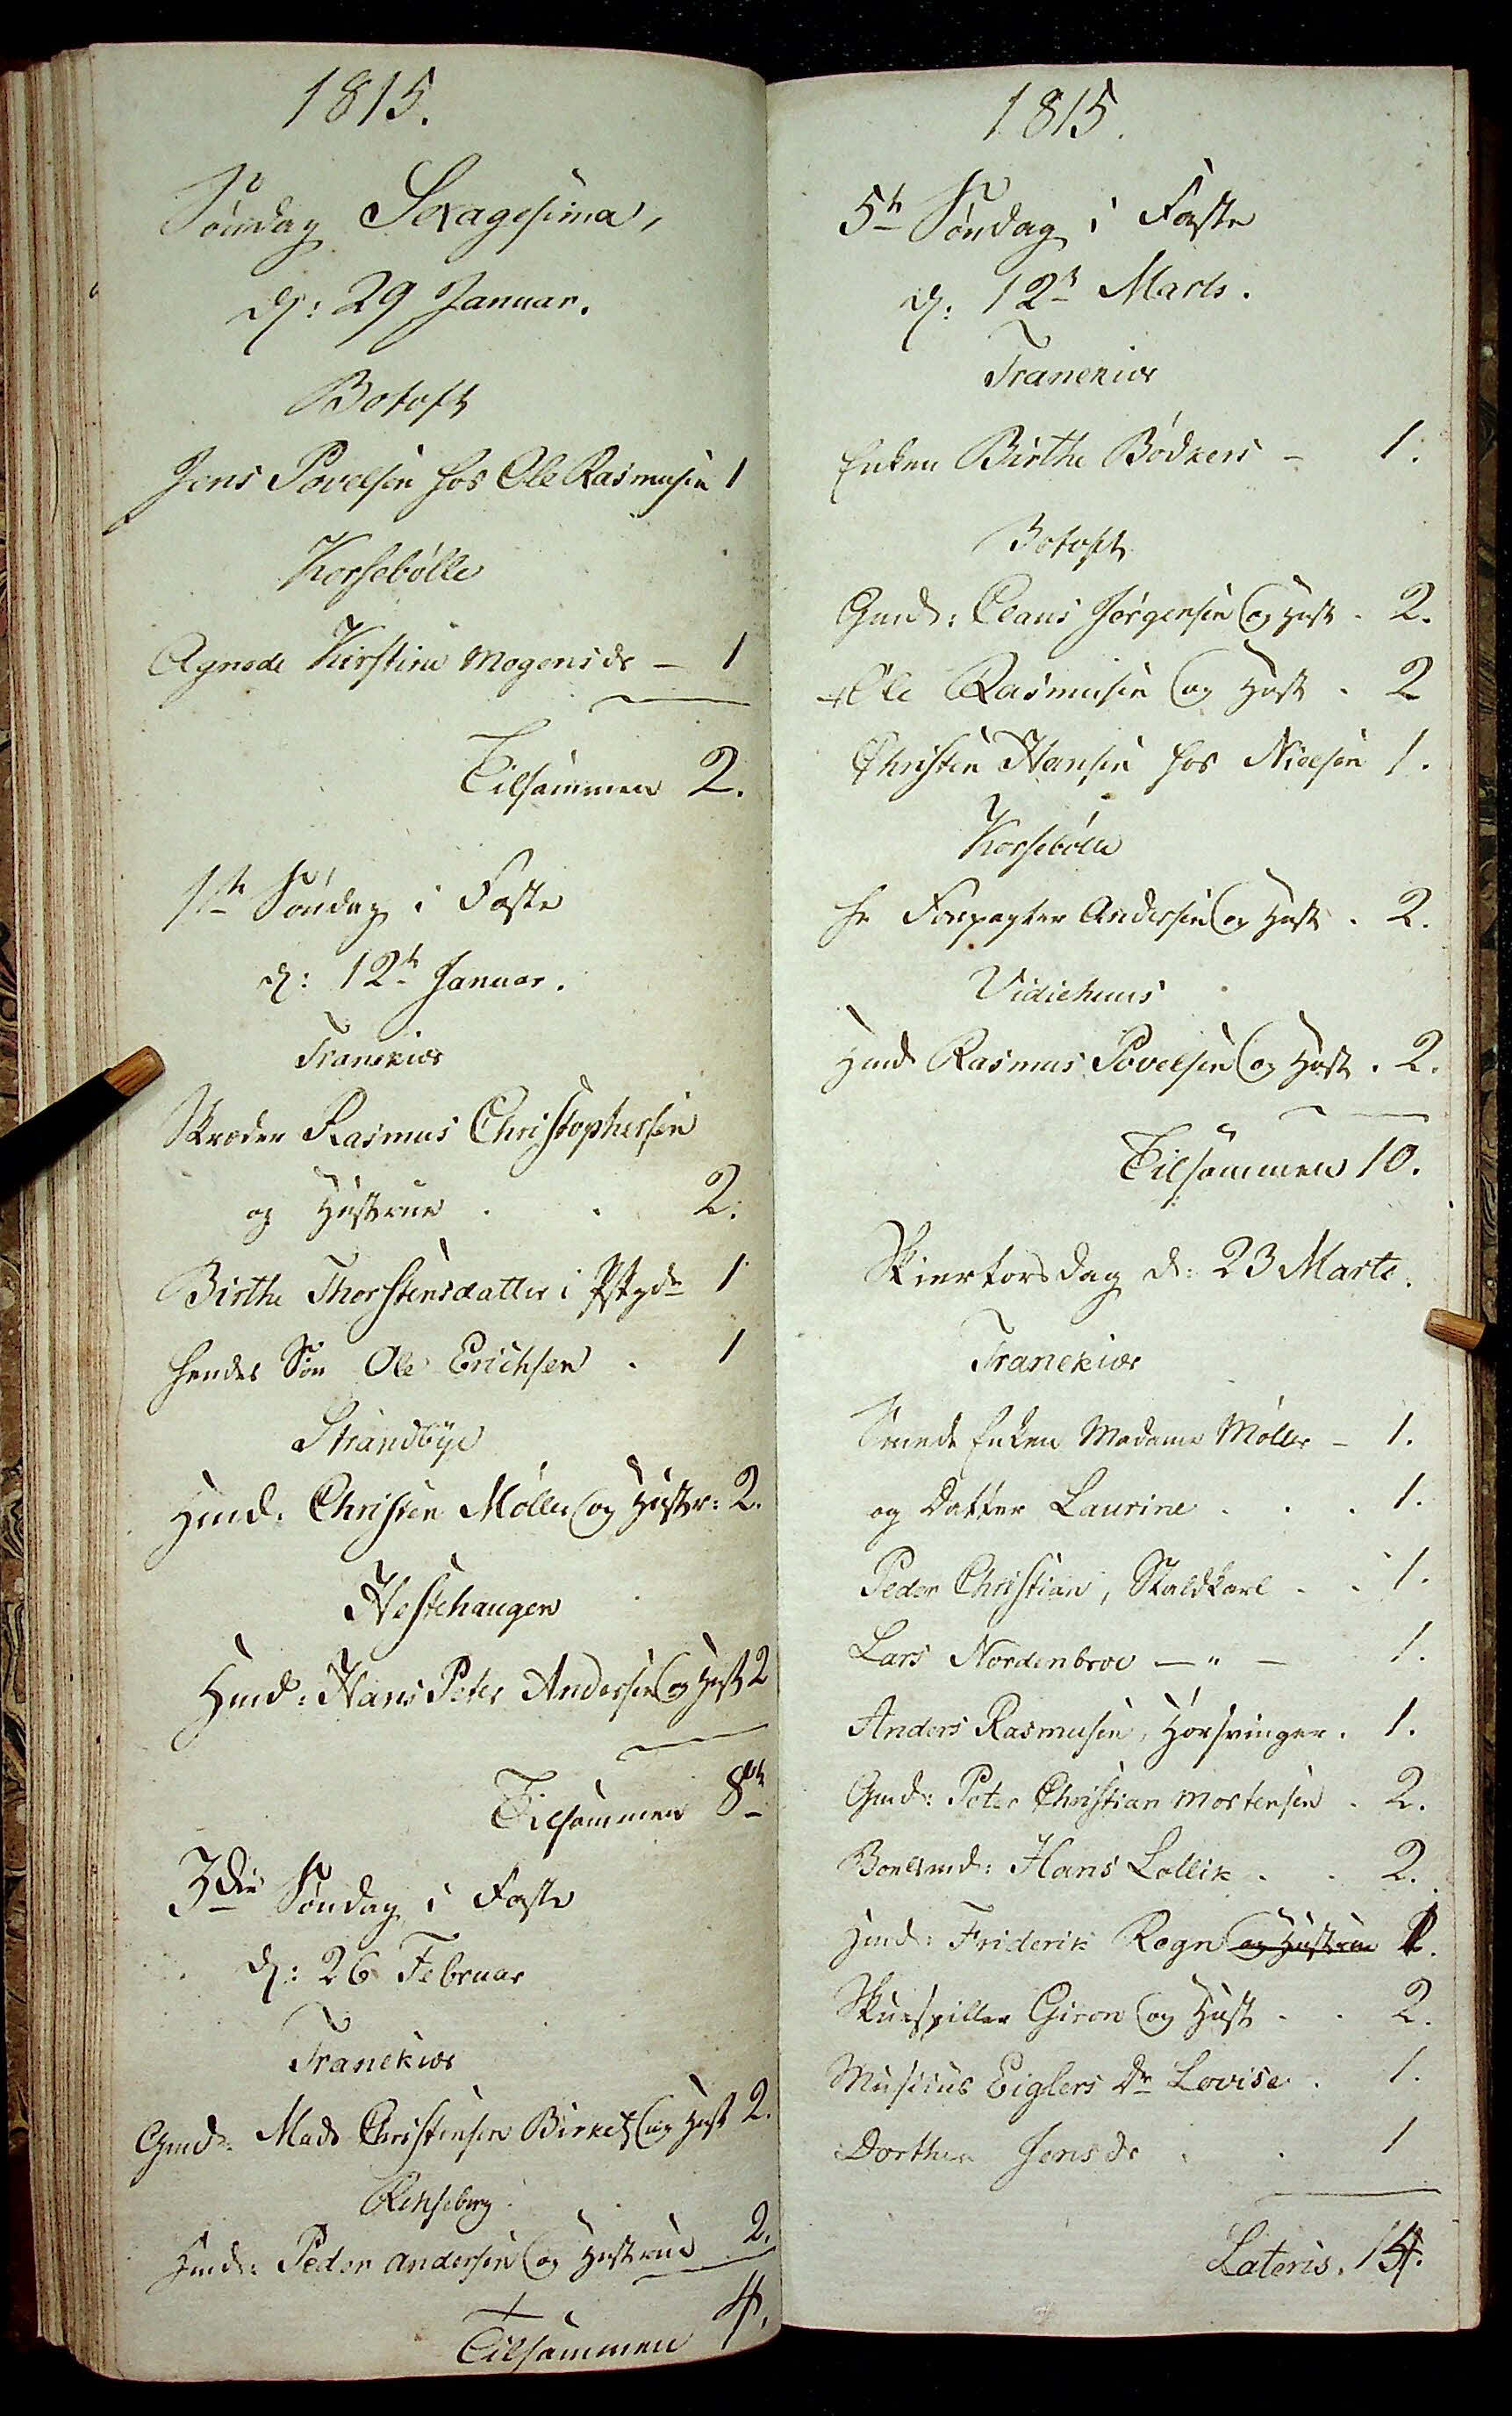1815.
Søndag Sexagesima.
d: 29 Januar.
Botoft
Jens Povelssen hos Ole Rasmusen 1
Korsebølle
Agnede Kirstine Mogensdr - 1
Tilsammen 2.
1ste Søndag i Faste
d: 12de Januar.
Tranekiær
Skræder Rasmus Christophersen
og Hustrue . . 2.
Birthe Thorstensdatter i Pstgdr 1
hendes Søn Ole Erichsen . 1
Strandbye
Hmd. Christen Møller og Hustr: 2.
Hestehaugen
Hmd. Hans Peter Andersen og Hust 2
Tilsamme 8##
3die Søndag i Faste
d: 26 Februar
Tranekiær
Gmd: Mads Christensen Birket og Hust 2
Renseberg
Hmd: Peder Andersens og Hustrue . 2
Tilsammen 4.
1815
5te Søndag i Faste
d: 12te Marts.
Tranekiær
Enken Birthe Bødkers - 1.
Bstoft.
Gmd: Claus Jørgensen og Hust . 2.
Ole Rasmusen og Hust . 2
Christen Hansen hos Nielsen 1.
Korsebølle
Hr Forpagter Andersen og Hust . 2.
Vidiehuus
Hmd Rasmus Povelsens og Hust . 2.
Tilsammen 10.
Skiertorsdag d: 23 Marts.
Tranekiær
Smede Enken Madame Mølle - 1.
og Datter Laurine . . . 1.
Peder Christian, Staldkarl . . 1.
Lars Nordenbroe -"- 1.
Anders Rasmusen Hørsvinger . 1.
Gmd: Peter Christian Mortensen . 2.
Boelsmd: Hans Lollik . . 2.
Hmd: Friderik Rogn og Hustrue 1.
Skuespiller Girone og Hust . . 2.
Musicus Eiglers Dr Lovise . 1.
Dorthe Jensdr . . 1
Lateris 14
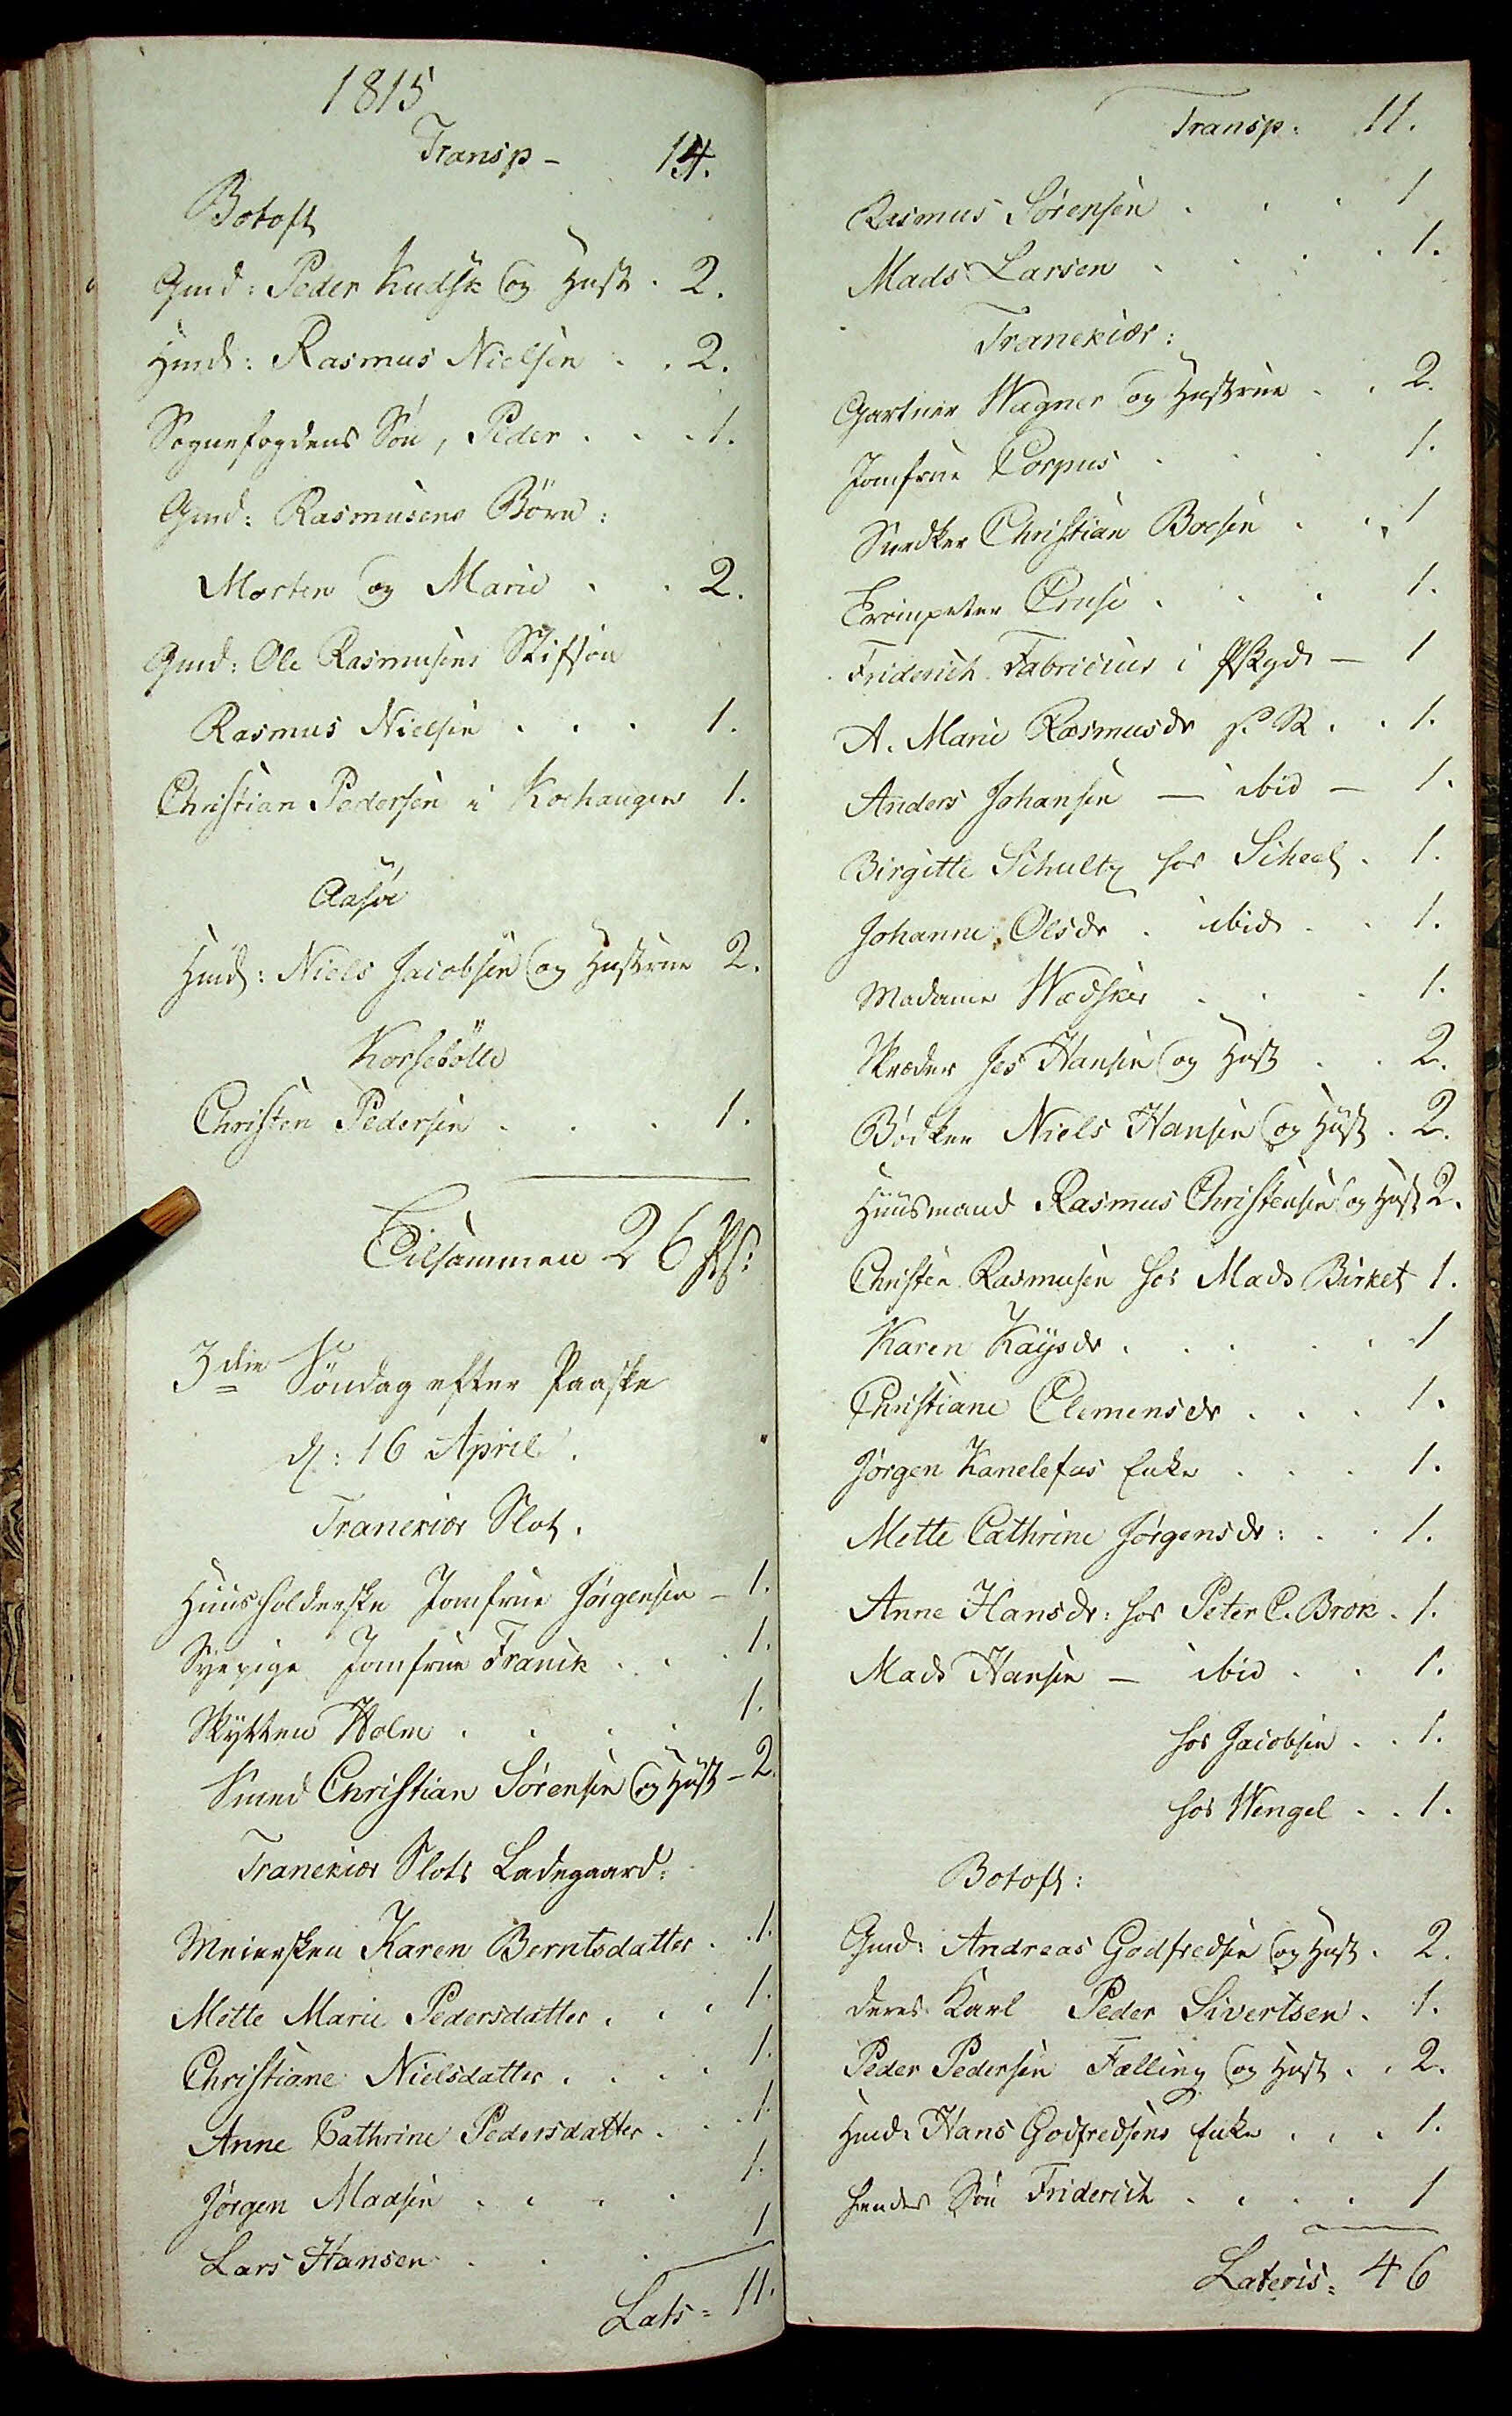1815
Transp - 14.
Botoft
Gmd: Peder Kudsk og Hust . 2.
Hmd: Rasmus Nielsen . . 2.
Sognefogdens Søn, Peder . . . 1.
Gmd: Rasmusens Børn:
Morten og Marie . . 2.
Gmd: Ole Rasmusens Stifsøn
Rasmus Nielsen . . . 1.
Christian Pedersen i Koehaugen 1.
Aasøe
Hmd: Niels Jacobsen og Hustrue 2.
Korsebølle
Christen Pedersen . . . 1.
Tilsammen 26 Ps:
3die Søndag efter Paaske
d: 16 April
Tranekiær Slot.
Huusholderske Jomfrue Jørgensen - 1.
Syepige Jomfrue Franck . . . 1.
Skytten Holm . . . . 1.
Smed Christian Sørensens og Hust - 2
Tranekiær Slots Ladegaard:
Meiersken Karen Berntsdatter . .1.
Mette Marie Pedersdatter . . . 1.
Christiane Nielsdatter . . . .1.
Anne Cathrine Pedersdatter . . . 1.
Jørgen Madsen . . . . 1.
Lars Hansen . . . 1
Lats= 11
Transp: 11.
Rasmus Sørensen . . . 1
Mads Larsen . . . . 1.
Tranekiær:
Gartner Wagner og Hustrue . . 2.
Jomfrue Corpus . . . 1.
Snedker Christian Boesen . . 1
Ervinpeter## Cense## . . . 1.
Friderich Fabricius i Pstgdr - 1
A. Marie Rasmusdr s. st . . 1.
Anders Johansen - ibid - 1.
Birgitte Schultz hos Scheel. 1.
Johanne Olsdr .  ibid . . 1.
Madame Vædsker . . . 1. 
Skræder Jes Hansen og Hust . . 2.
Bødker Niels Hansen og Hust . 2.
Huusmand Rasmus Christensens og Hust 2.
Christen Rasmusen hos Mads Birket 1.
Karen Kaysdr . . . . . . 1
Christiane Clemensdr . . . 1.
Jørgen Kanelefas Enke . . . 1.
Mette Cathrine Jørgensdr: . . 1.
Anne Hansdr: hos Peter C. Brok . 1.
Mads Hansen - ibid . . 1.
hos Jacobsen . . 1.
hos Wengel . . 1.
Botoft:
Gmd: Andreas Godfredsen og Hust . 2.
deres Karl Peder Sivertsen . 1.
Peder Pedersen Fælling og Hust . .  2.
Hmd Hans Godfredsens Enke . . . 1.
hendes Søn Friderich . . . . 1
Lateris= 46